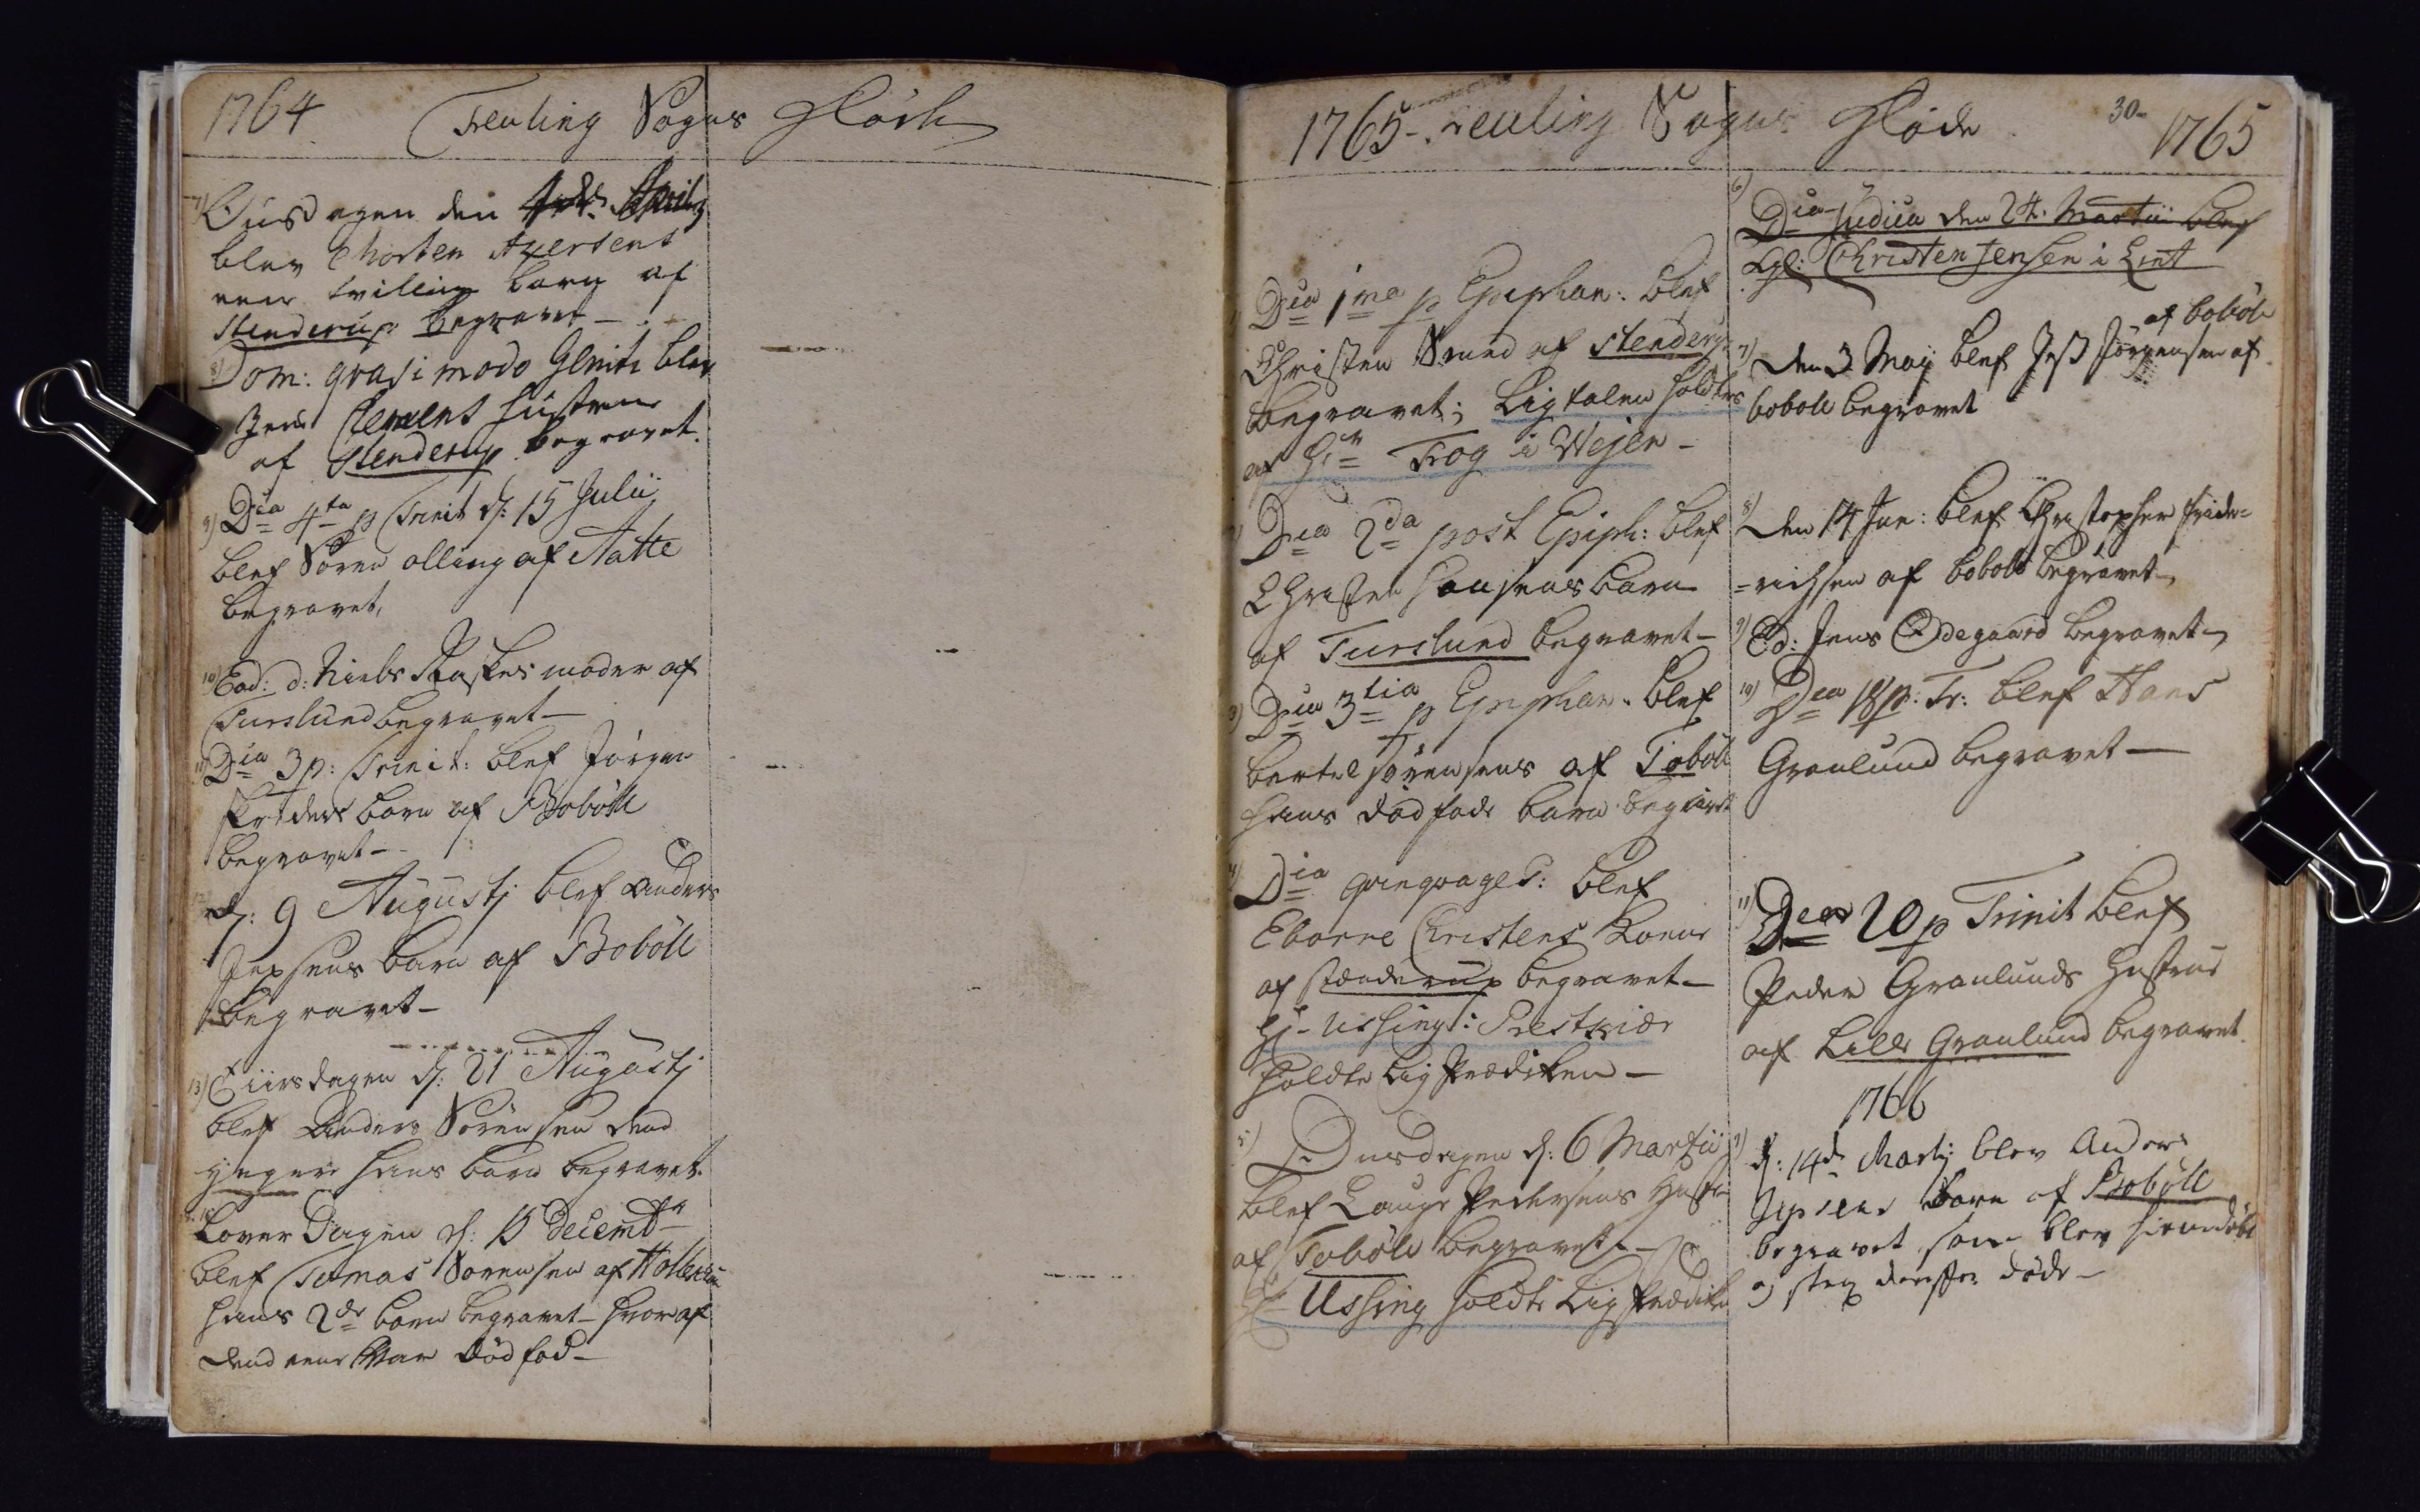1764
Feulings Sogns Døde
7) Onsdagen den ## ##
blev Morten Azersens
eene tvillinge barn af
Stenderup begravet -
8) Dom: Qvasimodo Geniti blev
Jens Clemens Hustrue
af Stenderup begravet.
9) Dca 4ta p. Trinit d: 15 Julii
blef Søren Olling af Aatte
begravet
10) Eod: d: Niels Raskes moder af
Tiirslund begravet -
11) Dca 3 p: Trinit: blef Jørgen
skreders Barn af Bobøll
begravet.
12) d: 9 Augusti blef Anders
Jepsens Barn af Bobøll
begravet -
13) Tiirsdagen d: 21 Augustj
blef Anders Sørensen den
yngere hans barn begravet.
##
Lover Dagen d: 15 Decembr
blef Tomas Sørensen af Holleschou
hans 2de born begravet hvor af
dend eene var dødfod -
1765 Feuling Sogns Døde
1) Dca 1ma p. Epiphan: Blef
Christen Smed af Stenderup.
begravet; Liigtalen holtes
af Hlr Fog ki Wejen -
2) Dca 2da post Epiph: Blef
Christen Hansens Barn
af Tiirslund begravet-
3) Dca 3tia Epiphan: Blef
Bertel Sørensens af Tobøll
hans dødfode barn begravet
4)
Dca Qvinqvages: blef
Eborre Christens Koene
af Stænderup begravet -
Hlr Ussing: Prestkiær
holdte Liig Prædiken -
5) Onsdagen d: 6 Martii
blef Lauge Pedersens Hustr:
af Tobøll begravet. -
Hlr Ussing holdte Liig Prædiken
30.
1765
6)
Dca Judica den 24 Martii blef
Gl: Christen Jensen i L##et
af bobøll
7) Den 3 Maij blef Jess Jørgensen af
boboll begravet
8)
Den 14 Jan: blef Christopher Fride¬
= richsen af boboll begravet -
9)
E:d: Jens Ødegaard begravet -
10)
Dca18 p. Tr: blef Hans
Graulund begravet -
11)
Dca 20 p Trinit blef
Peder Graulunds Hustrue
af Lille Graulund begravet
1766
1)
d: 14de Martij blev Anders
Jepsens barn af Bobøll
begravet, som blev hiemdøbt
opg strax derefter døde -
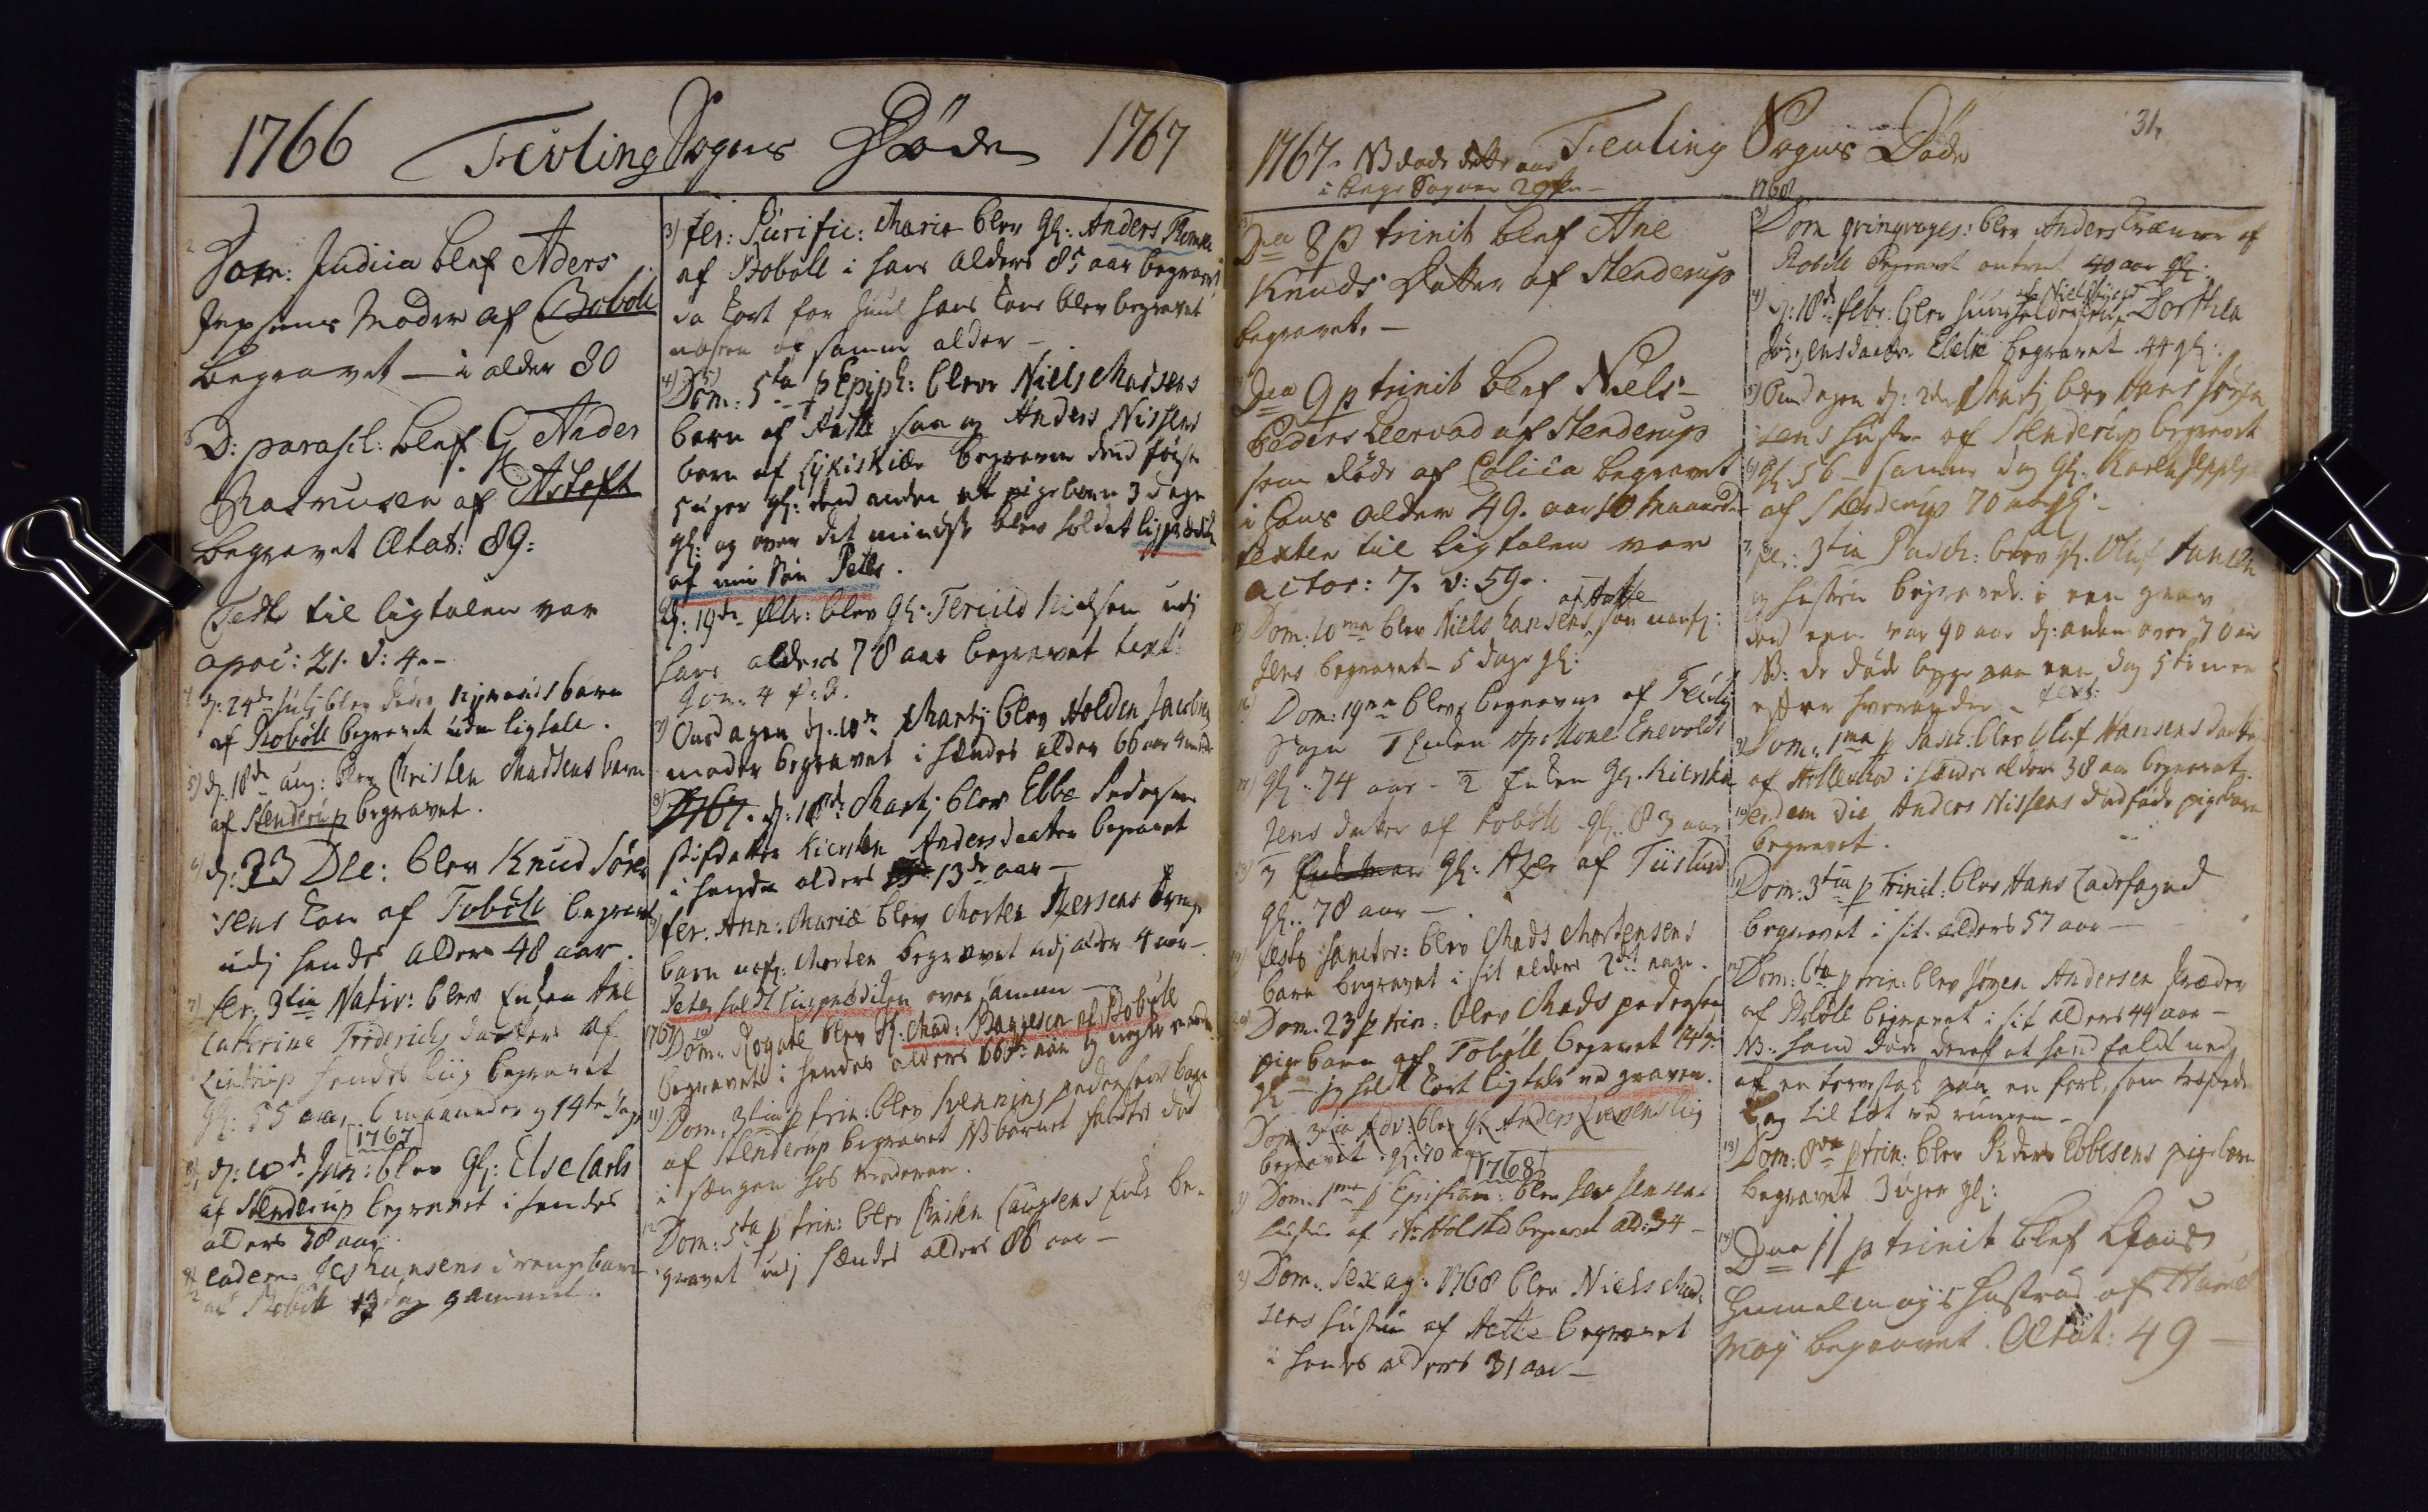1766 Fesling Sogns Døde
2
Dom: Judica blef Aders
Jepsens Moder af Boboll
begravet - i alder 80
3
D: Parasch: blef Ander
Rasmussen af Astoft
begravet Ætat: 89:
Text til Liigtolen var
apoc: 21. d: 4.-
4
d. 24de Juli blev Peder ##s barn
af Bobøll begravet uden ligtale
5) d: 18de Aug: blev Christen Madsens barn
af Stenderup begravet.
6) d 23 Dec: blev Knud Søren
sens Kone af Tobøll begravet
udj hands alders 48 aar.
7) Fer. 3tia Nativ: blev Enken Ane
Cathrine Friderichs Datter af
Lintrup hendes Liig begravet
gl: 55 aar 6 maaneder og 14te dage
[1767]
8) 1 d: 10de Jan: blev gl: Else Carls
af Stenderup begravet i hendes
alders 78 aar
9) 2 Eodem Jes hansens drengebarn
af Bobøll 13 dage gammel.
1767
3) Fer: Purific: Marie blev gl: Anders Thomsen
af Bobøll i hans alders 85 Aar begravet
da kort for Juul hans Kone blev begravet
næsten af samme alder
4) 5)
Dom: 5ta Epiph: blev Niels Madsens
barn af Aatte saa og Anders Nissens
barn af Lykiskiær begravne den første
5 uger gl. dend anden at pigebarn 3 dage
gl: og over det mindste blev holdet Ligprædiken
af min Søn Peter
6)
d: 19de Febr: blev gl: Tercild Nielsen udj
hans alders 78 Aar begravet text:
J##: 4 f: 3.
Onsdagen d: 18de Martij blev Holden Jacobs
moder begravet i hendes alder 66 Aar gammel.
8)
1767. d: 18de Martj blev Ebbe Pedersens
stifdatter Kiersten Andersdatter begravet.
i hende alders ## 13de Aar
Fer: Ann: Mariæ blev Morten Azersens Drenge
barn nafl: Morten begravet udj alder 4 aar .
Peter holdt Liigprædiken over hannem
10)
1767
Dom: Rogate blev Sl: Mad: Baggesen ag Bobøll
begravet i hendes alders 66## aar og nogle ##
11).
Dom; 3tia  p Trin: bleven Svenning pedersens barn
af Stenderup begravet NB barnet fandtes død
i sængen hos Mederen
12
Dom: 5ta p Trin: blev Chrine Laugsens Enke be¬
gravet udj hændes alders 86 aar
1767.
NB dode dette aar
i bege Sogne 29 Pe
13)
Dca 8 p Trinit blef Ane
Knuds Datter af Stenderup
begravet. -
14) Dca 9 p Trinit blef Niels
Peders Leervad af Stenderup
som døde af Colica begravet
i hans alder 49. aar 10 maaneder.
Texten til Lig talen var
actor: 7. v: 59.
af Aatte
15) Dom: 10ma blev Niels Hansens ^ søn navfl:
Jens begravet - 5 dage gl:
16)
Dom: 19## blev begravne af Feuling
Sogn Enken 1 Enken Apollone Enevolds
17)
gl: 74 aar -, 2 Enken gl: Kiersten
Jens Datter af Tobøll gl: 83 Aar
18)  3 Enkeman gl: Azer af Tiislund
gl: 78 aar
19) Festo Sanctor: blev Mads Mortensens
barn begravet i sit alders 2det aar.
20)
Dom. 23 p Trin: blev Mads Pedersen
pigebarn af Tobøll begravet ##
gl ##  jg kolrt Ligtale ved graven.
Dom 3tia Adv: blev gl: Anders Iversens Liig
begravet gl: 70 Aar
[1768]
1) Dom: 1ma Epiphan: blev Jens Jensens
hustrue af st: Holsted begravet ald: 34
2) Dom: Sexag: 1768 blev Niels Mad¬
sens hustrue af Aatte begravet
i hendes alders 31 Aar -
Feuling Sogns Døde
31.
1768
3)
Dom Qvinqvages: blev Anders ## af
Bobøll begravet omtrent 40 aar gl.
af Nielsbyegd
4)
d: 18de Febr: blev huusholdersken ^ Dorthea
Jørgensdatter Ebeløe begravet 44 gl:
5) Onsdagen d: 2den Martj blev Hans Jørgen
sens Hustru af Stenderup begravet
6) gl: 56 samme Dag gl: Karen Jeppes
af Stenderup 70 aar gl
7, 8, 
d: 3tia Pasch: blev gl. Oluf Hansen
og hustru begravet i een grav
den ene var 90 aar d: anden vare 70 aar
NB: de døde begge paa een dag 5 timer
efter hverandre
text:
9) Dom: 1ma p Pasch: blev Oluf Hansens Datter
af Holleskov hendes alders 38 aar begravet
10) eodem Die Anders Nilsens dødføde pige barn
begravet
11) Dom: 3tia p Trinit: blev Hans Ladefoged
begravet i sit alders 57 Aar -
12)
Dom: 6ta p Trin: blev Jørgen Andersen skræder
af Bobøll begravet i sit alders 44 Aar -
NB: hand døde deraf at hand faldt ned
af en torvestak paa en fork, som trøstede
bag til tøt ved rumpen
13)
Dom: 8va p Trin: blev Peder Ebbesens pigebarn
begravet 3 uger gl:
14)
Dca: 11 p Trinit blef Claus
H##s Hustrue af Hume##
Moy begravet ætat: 49